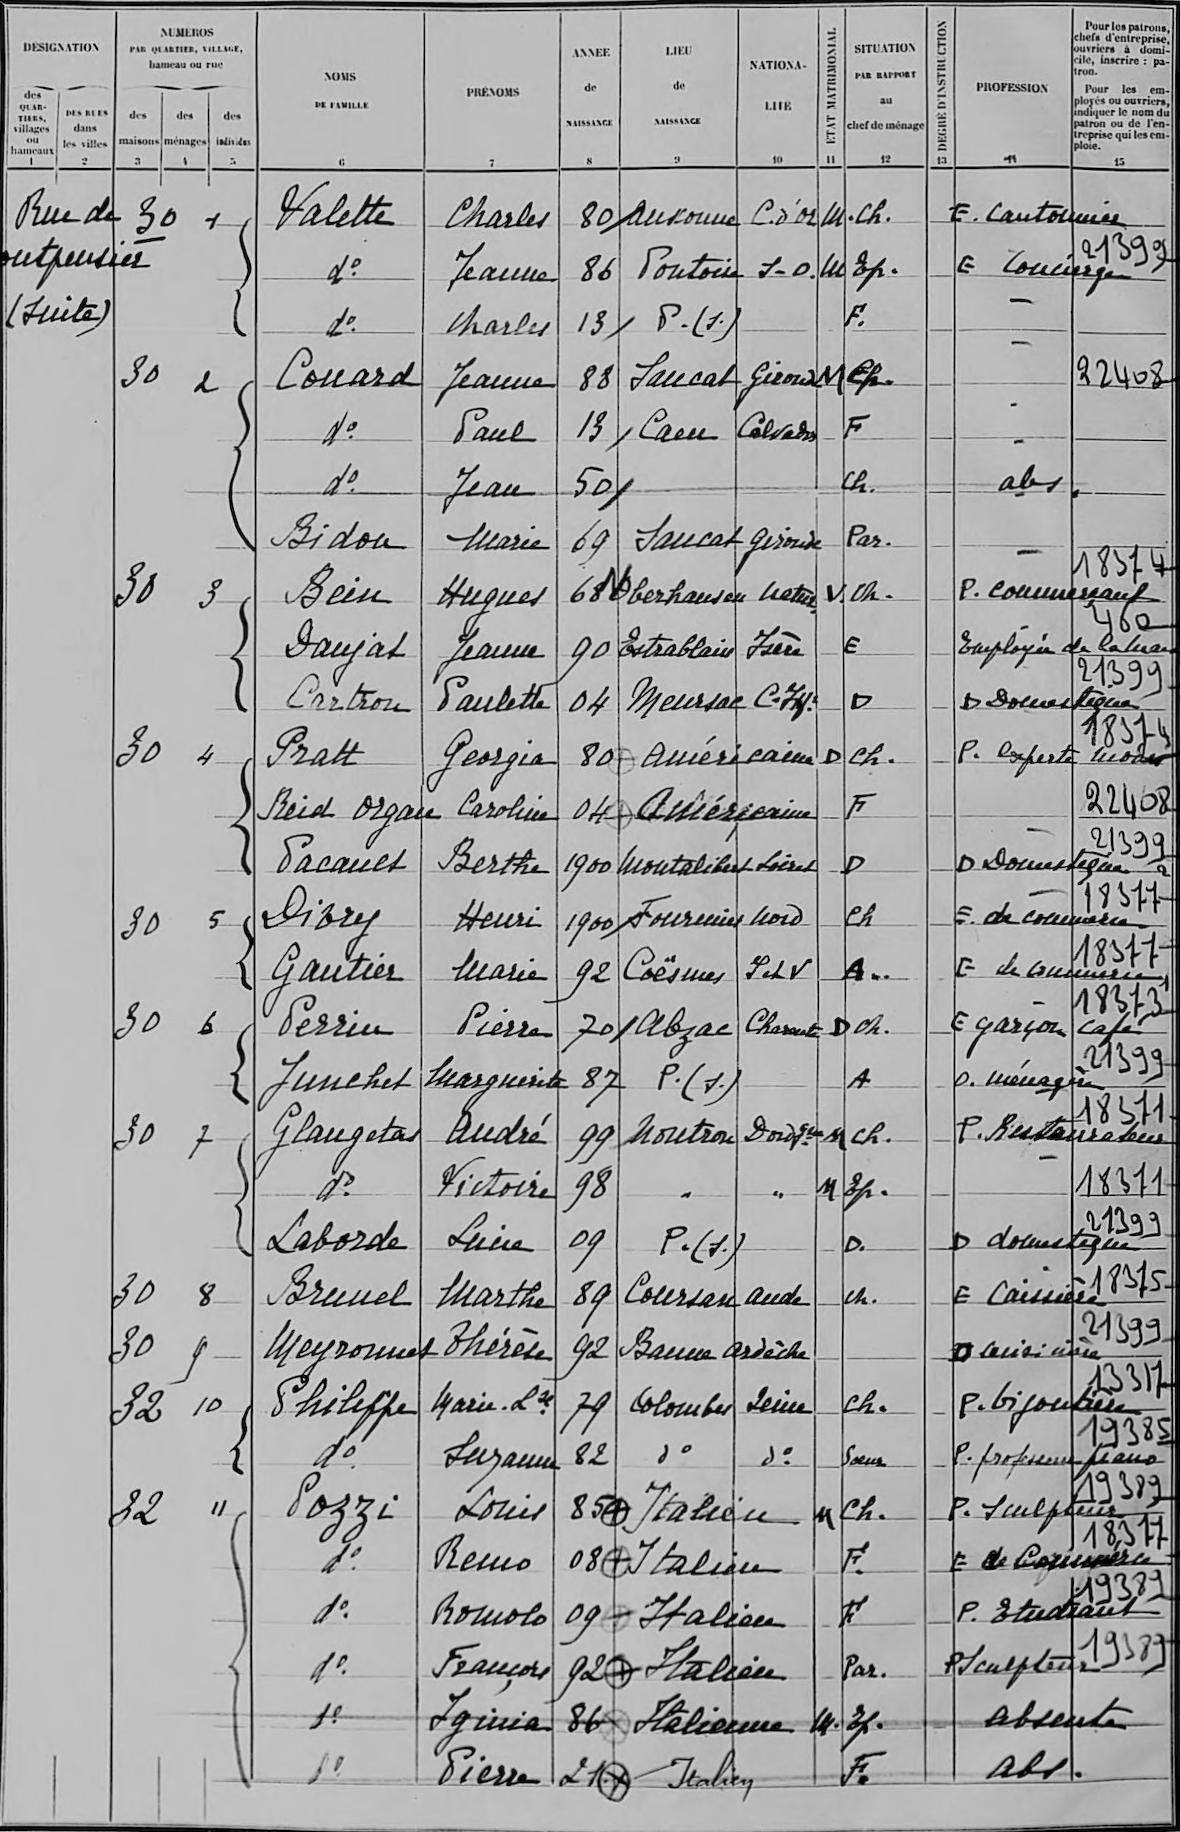Valette/Charles/80/Auxonne/C d'or/m /Ch /¤/E cantonnier/¤
d°/Jeanne/86/Pontoise/S-O/m/Ep /¤/E Concierge/?21399
d°/Charles/13/P (s )/¤/¤/F /¤/¤/¤
Couard/Jeanne/88/Saucat/Gironde/M/Ep /¤/¤/22408
d°/Paul/13/Caen/Calvados/¤/F/¤/¤/¤
d°/Jean/50/¤/¤/¤/Ch /¤/abs/¤
Bidou/Marie/69/Saucat/Gironde/¤/Par /¤/¤/¤
Bein/Hugues/68/Oberhausen/natur/V /Ch /¤/P commerçant/?18374
Daujat/Jeanne/90/Estrablain/Isère/¤/E/¤/Employée de la maison/?460
Cartrou/Paulette/04/Meursac/C Inf/¤/D/¤/D Domestique/?21399
Pratt/Georgia/80/Américaine/¤/D/Ch /¤/P Experte mode/?18374
Reid Organ/Caroline/04/Américaine/¤/¤/F/¤/¤/22408
Pacauet/Berthe/1900/Montalibert/Loiret/¤/D/¤/D Domestique/?21399
Dibry/Henri/1900/Fourmies/Nord/¤/Ch/¤/E de commerce/?18377
Gautier/Marie/92/Coësmes/I et V/¤/A /¤/E de commerce/?18377
Perrin/Pierre/70/Abzac/Charente/D/Ch /¤/E garçon café/?18373
Junchet/Marguerite/87/P (s)/¤/¤/A/¤/D ménagère/?21399
Glaugetas/André/99/Nontron/Dordgne/M /Ch /¤/P restaurateur/?18371
d°/Victoire/98/¤/¤/M /Ep /¤/¤/18371
Laborde/Lucie/09/P (s )/¤/¤/D /¤/D domestique/?21399
Brunel/Marthe/89/Coursan/aude/¤/ch /¤/E caissièr/18375
Meyronnet/Thérèse/92/Banne/Ardèche/¤/¤/¤/o cuisini/21399
Philippe/Marie-Lse/79/Colombes/Seine/¤/Ch /¤/P bijoutière/?13317
d°/Suzanne/82/d°/d°/¤/Soeur/¤/P professeur piano/?19385
Pozzi/Louis/85/Italien/¤/m /Ch/¤/P sculpteur/?19389
d°/Remo/08/Italien/¤/¤/F /¤/E de commerce/?18377
d°/Romolo/09/Italien/¤/¤/F/¤/P Etudiant/?19389
d°/François/92/Italien/¤/¤/Par /¤/P sculpteur/19389
d°/Iginia/86/Italienne/¤/M /Ep /¤/Absente/¤
d°/Pierre/21/Italien/¤/¤/F /¤/abs /¤
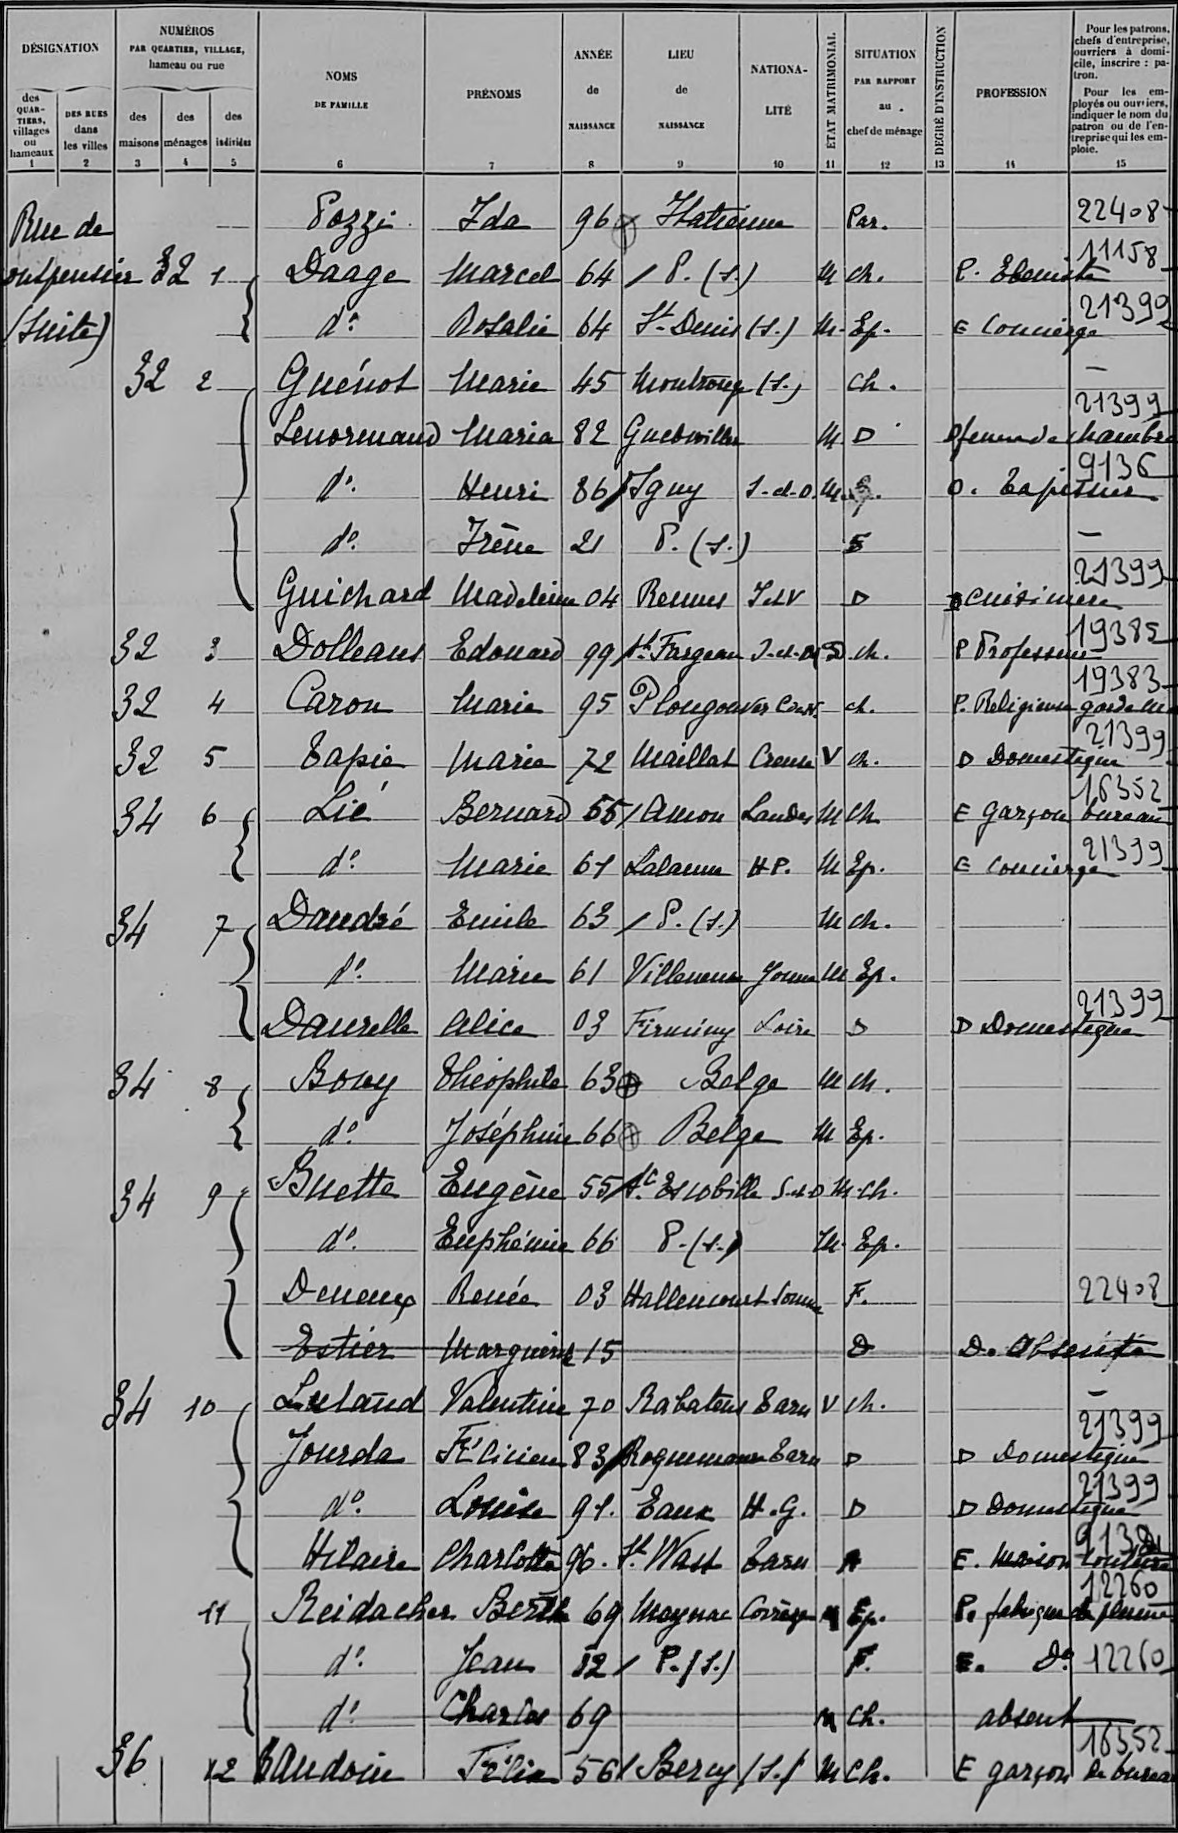Pozzi/Ida/96/Italienne/¤/¤/Par/¤/¤/22408
Darge/Marcel/64/P (s)/¤/m/ch /¤/P Ebeniste/?11158
d°/Rosalie/64/St Denis (s )/¤/m /Ep /¤/E Concierge/?21399
Guénot/Marie/45/Montrouge (s )/¤/¤/Ch /¤/¤/¤
Lenormand/Maria/82/Guesbwiller/¤/m/D/¤/D femme de chambre/?21399
d°/Henri/86/Igny/S et O /m/¤/¤/O tapissier/?9136
d°/Irène/21/P (s) /¤/¤/¤/¤/¤/¤
Guichard/Madeleine/04/Rennes/I et V /¤/D/¤/D cuisinière/?21399
Dolleaus/Edouard/99/St Fargeau/I et V /D/ch /¤/P Professeur/?19385
Caron/Marie/95/Plougonver/C du N /¤/ch /¤/P Religieuse garde m/?19383
Tapie/Marie/72/Maillat/Creuse/V/ch /¤/D domestique/?21399
Lié/Bernard/55/Amou/Landes/M/Ch/¤/E garçon bureau/?16352
d°/Marie/61/Lalanne/H P /M/Ep /¤/E concierge/?21399
Dandré/Emile/63/P (s )/¤/M/ch /¤/¤/¤
d°/Marie/61/Villeneuve/Yonne/M/Ep /¤/¤/¤
Daurelle/Alice/03/Firminy/Loire/¤/D/¤/D Domestique/?21399
Bovy/Théophile/63/Belge/¤/m/ch /¤/¤/¤
d°/Joséphine/66/Belge/¤/M /Ep /¤/¤/¤
Buette/Eugène/55/Sc Escobille/S et O/M /Ch /¤/¤/¤
d°/Euphénie/66/P (s )/¤/M /Ep /¤/¤/¤
Deneux/Renée/03/Hallencourt/Somme/¤/F /¤/¤/22408
Estier/Marguerite/15/¤/¤/¤/D/¤/D absente/¤
Lutaud/Valentine/70/Rabatens/Tarn/V/ch /¤/¤/¤
Jourda/Félicien/83/Roquemaure/Tarn/¤/D/¤/D Domestique/?21399
d°/Louise/91/Eaux/H G /¤/D/¤/D domestique/?21399
Hilaire/Charlotte/96/St Wast/Tarn/¤/A/¤/E maison/couture?9132
Reidacher/Berthe/69/Moyssac/Corrèze/M /Ep /¤/P fabrique de plume/?12260
d°/Jean/12/P (s )/¤/¤/F /¤/E d°/12260
d°/Charles/69/¤/¤/M /Ch /¤/absent/¤
Baudoin/Félix/56/Bercy/:S :/M/Ch /¤/E garçon de bureau/?16352